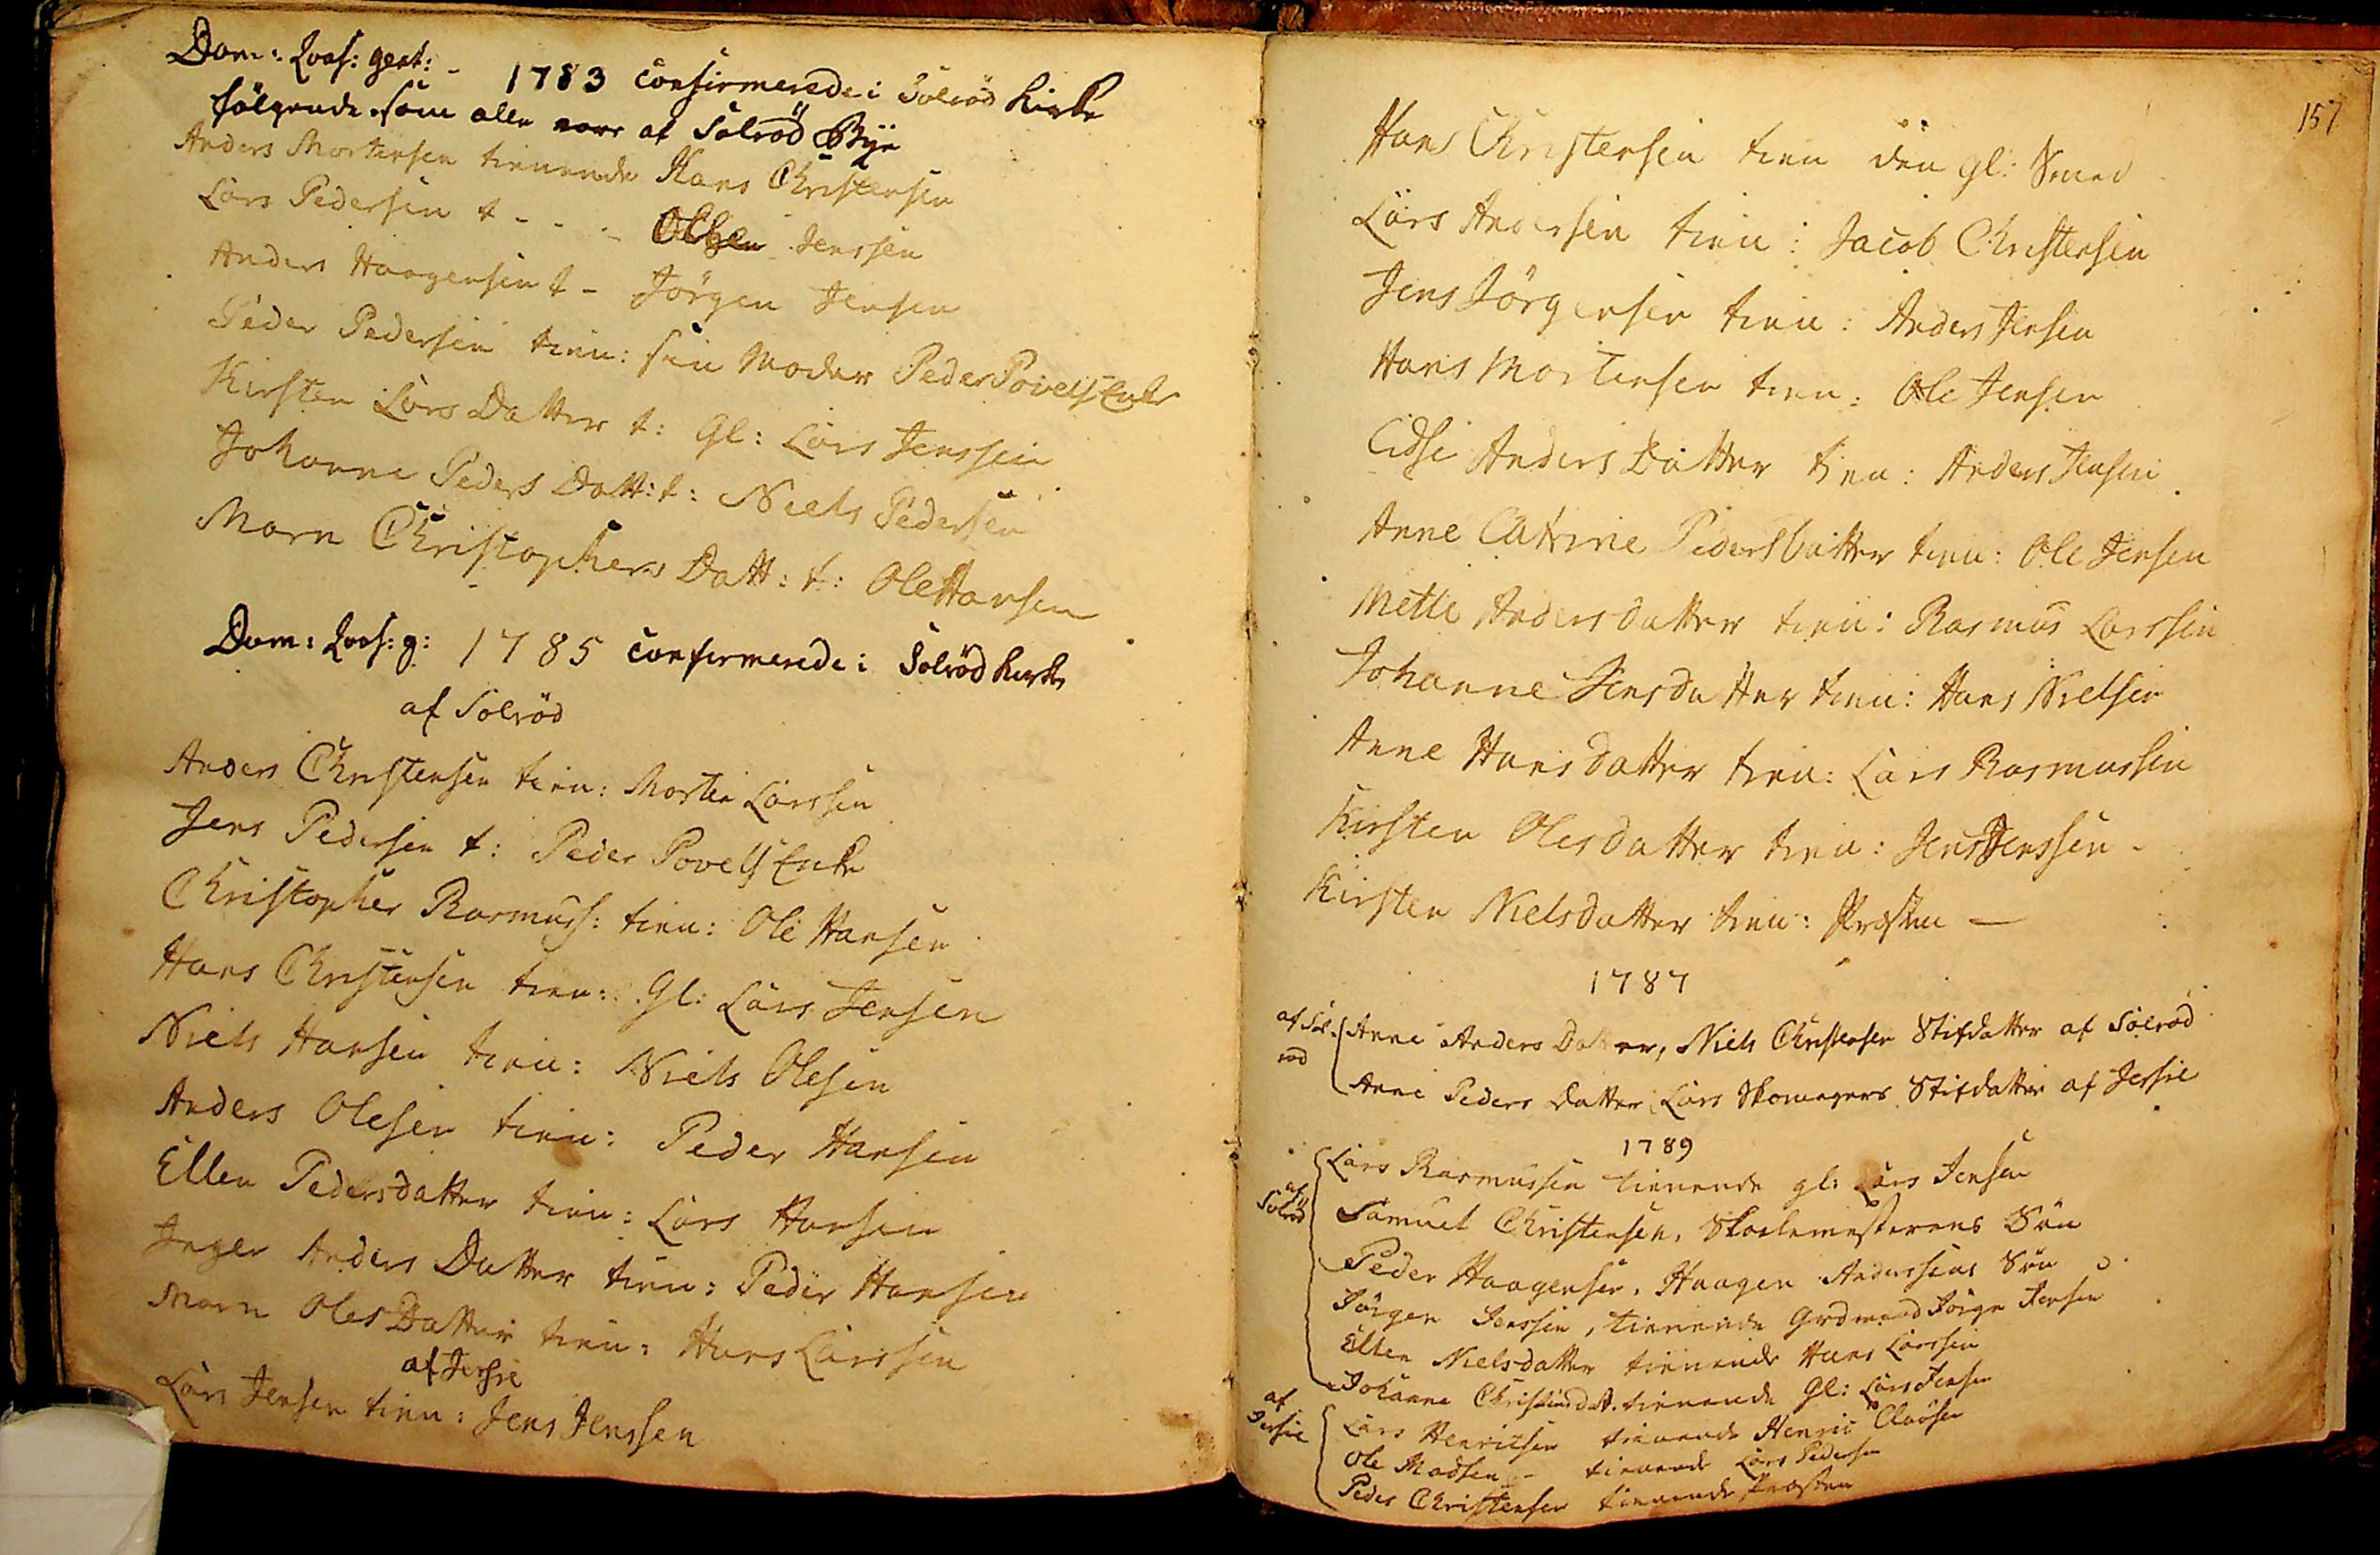Dom: Qvas: gent: - 1783 Confirmerede i Solrød Kirke
følgende som alle vare af Solrød Bye
Anders Mortensen tienende Hans Christensen
Lars Pedersen t - - - Ol## Jenssen
Anders Haagensen t - Jørgen Jensen
Peder Pedersen tien: sin Moder Peder Povels Enke
Kirsten Lars Datter t: Gl: Lars Jenssen
Johanne Peders Datt: t: Niels Pedersen
Marn Christophers Datt: t: Ole Hansen
Dom: qvas:g: 1785 Confirmerede i Solrød Kirke
af Solrød
Anders Christensen tien: Morten Larssen
Jens Pedersen t: Peder Povels Enke
Christopher Rasmuss: tien: Ole Hansen
Hans Christensen tien: Gl: Lars Jensen
Niels Hansen tien: Niels Olesen
Anders Olesen tien: Peder Hansen
Ellen Pedersdatter tien: Lars Hansen
Inger Anders Datter tien: Peder Hansen
Marn Oles Datter tien: Hans Larssen
af Jersie
Lars Jensen tien: Jens Jenssen
157
Hans Christensen tien: den gl: Smed
Lars Andersen tien: Jacob Christensen
Jens Jørgensen tien: Anders Jensen
Hans Mortensen tien: Ole Jensen
Cidse Anders Datter tien: Anders Jensen
Anne Catrine Pedersdatter tien: Ole Jensen
Mette Andersdatter tien: Rasmus Larssen
Johanne Jensdatter tien: Hans Nielsen
Anne Hansdatter tien: Lars Rasmussen
Kirsten Olesdatter tien: Jens Jenssen
Kirsten Nielsdatter tien: Præsten
1787
af Sol¬
rød
Anne Anders Datter, Niels Christensen Stifdatter af Solrød
Anne Peders Datter, Lars Skomagers Stifdatter af Jersie
1789
af
Solrød
Lars Rasmussen tienende gl: Lars Jensen
Samuel Christensen, Skolemesterens Søn
Peder Haagensen, Haagen Andersens Søn
Jørgen Jenssen, tienende Grdmand Jørgn Jensen
Ellen Nielsdatter tienende Hans Larssen
Johanne Christensdatt. tienende gl: Lars Jensen
af
Solrød
Lars Henricsen tienende Henric Clausen
Ole Madsen - tienende Lars Pedersen
Peder Christensen tienende Præsten 
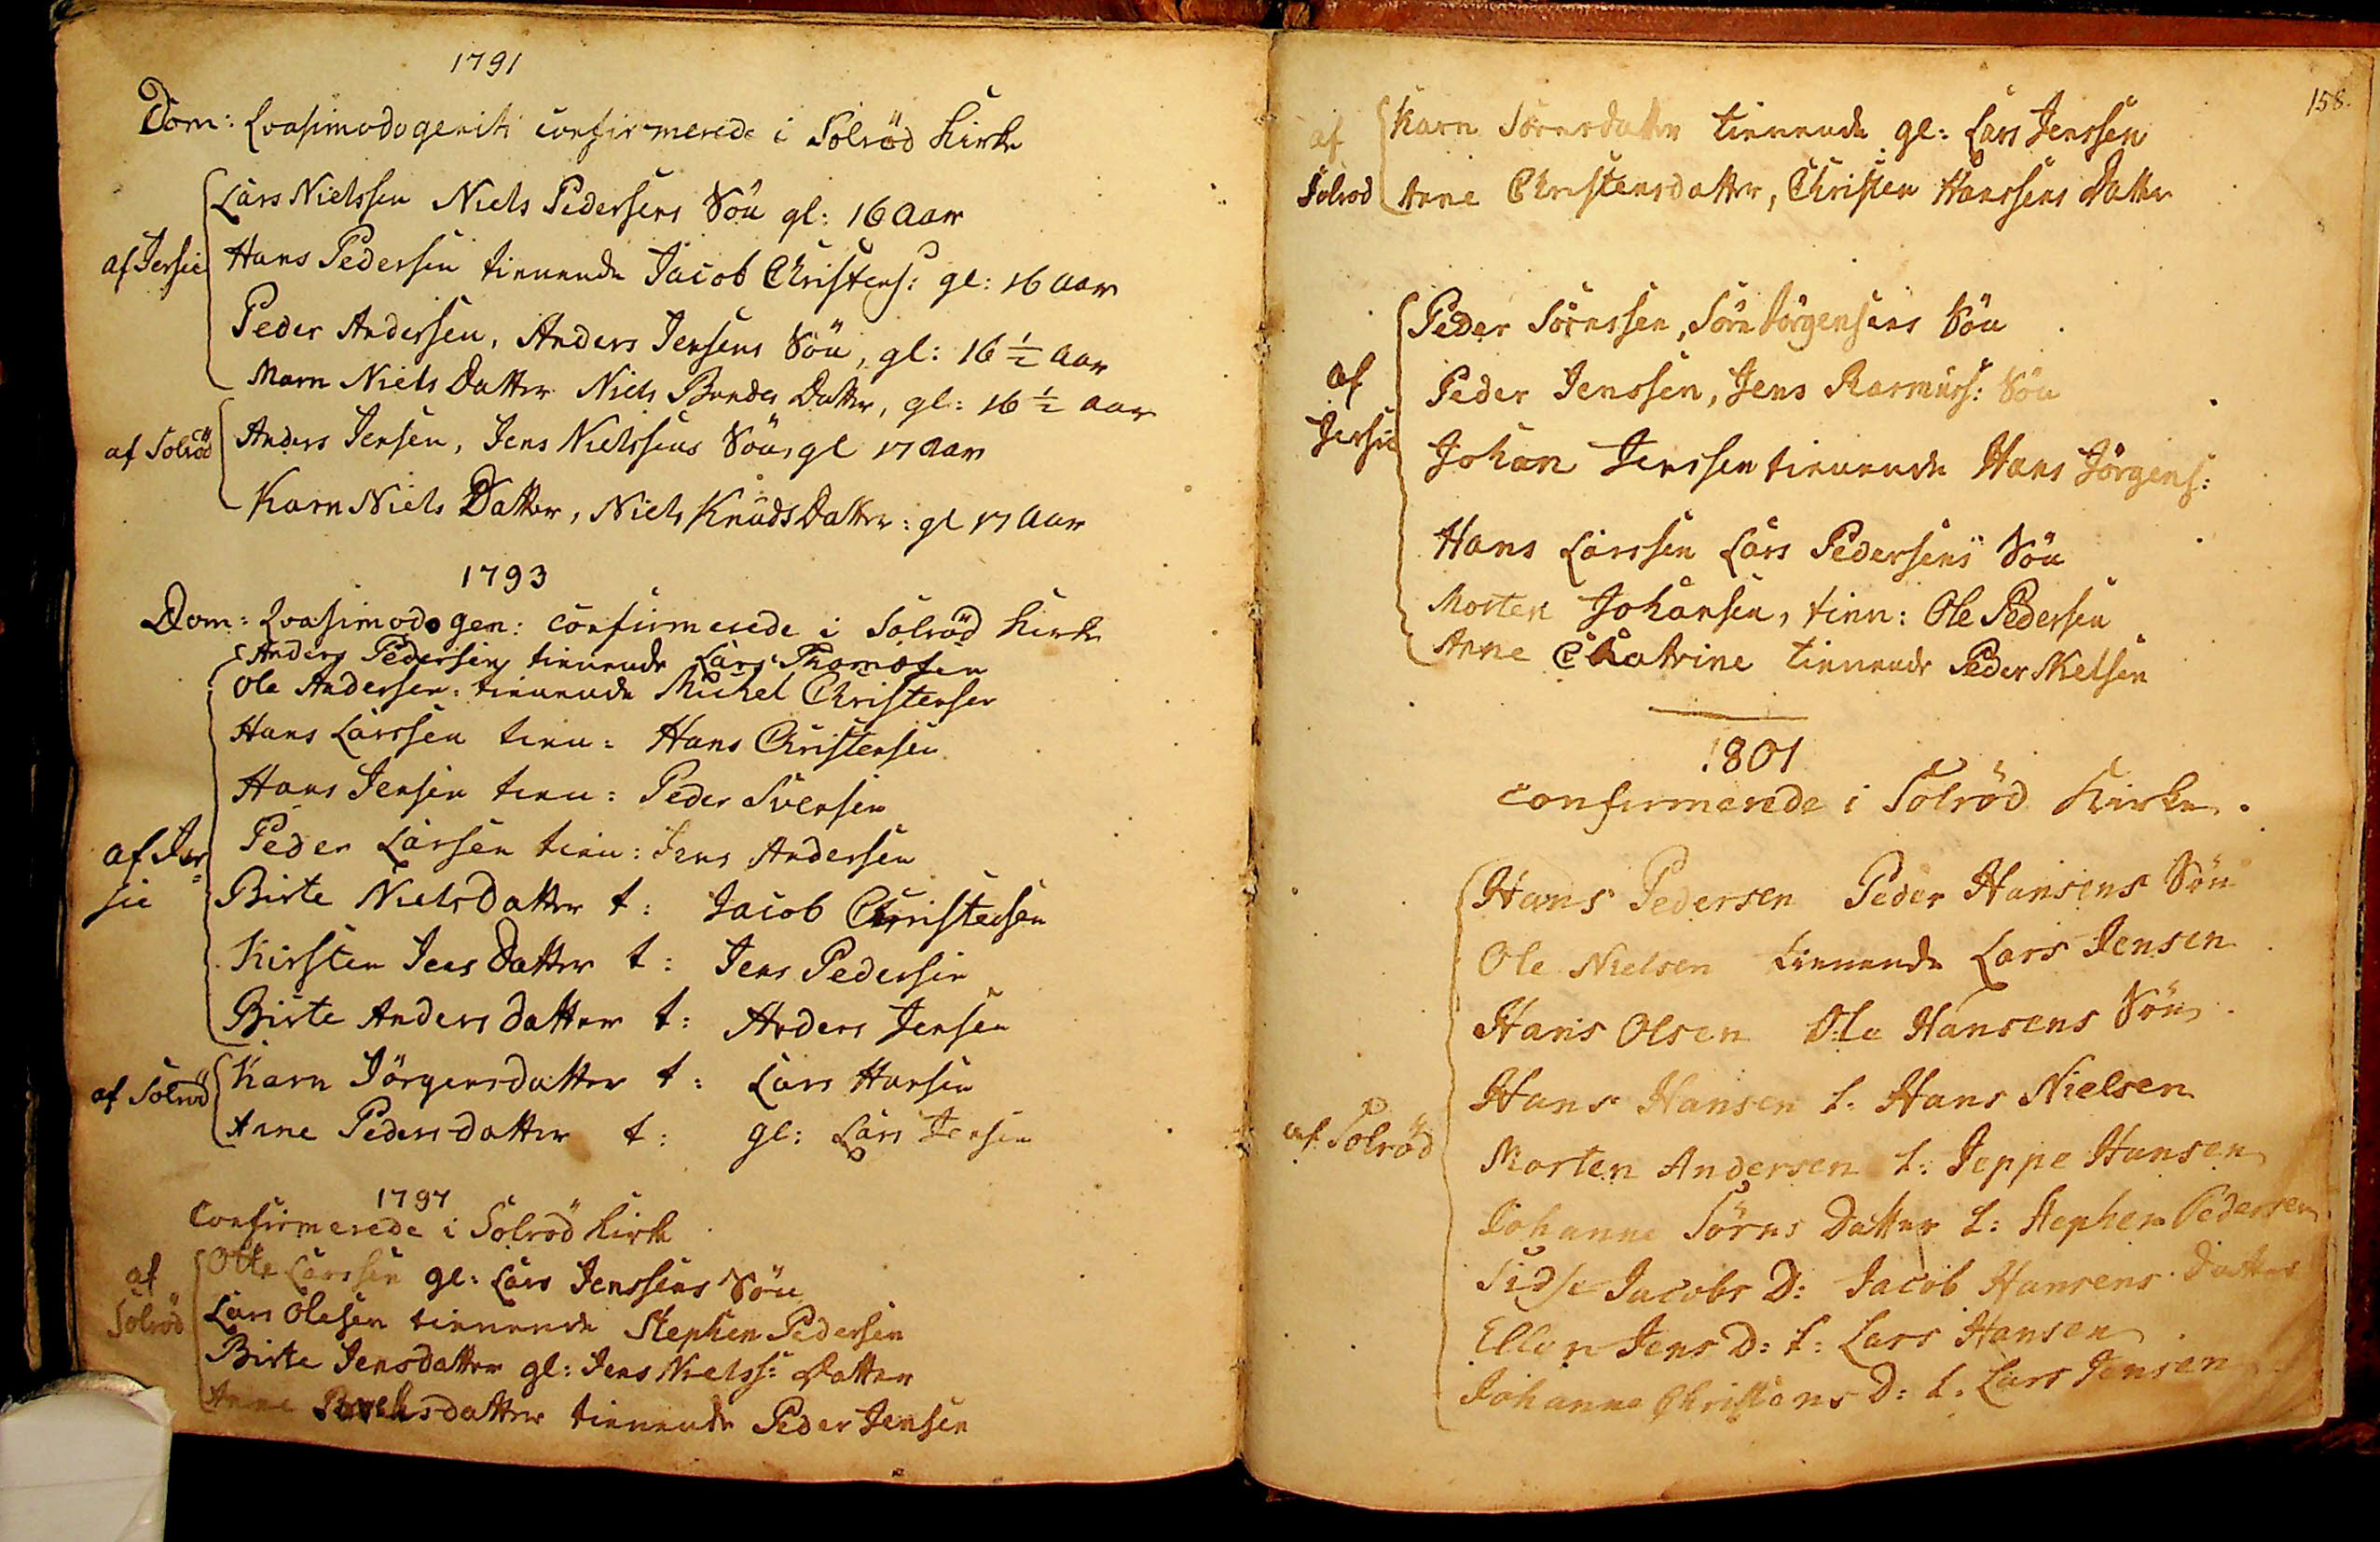1791
Dom: Qvasimodo geniti Confirmerede i Solrød Kirke
Lars Nielssen Niels Pedersens Søn gl: 16 Aar
af Jersie Hans Pedersen tienende Jacob Christens: gl: 16 Aar
Peder Andersen, Anders Jensens Søn, gl: 16½ Aar
Marn Niels Datter Niels Bondes Datter, gl: 16½ Aar
af Solrød Anders Jensen, Jens Nielssens Søn, gl 17 Aar
Karn Niels Datter, Niels Knuds Datter: gl 17 Aar
1793
af Jer¬
sie
Dom: Qvasimodo Gen: Confirmerede i Solrød Kirke
Anders Pedersen, tienende Lars Thomæsen
Ole Andersen, tienende Michel Christensen
Hans Larssen tien: Hans Christensen
Hans Jensen tien: Peder Svensen
Peder Larsen tien: Jens Andersen
Birte Niels Datter t: Jacob Christensen
Kirsten Jens Datter t: Jens Pedersen
Birte Anders Datter t: Anders Jensen
af Solrød Karn Jørgensdatter t: Lars Hansen
Anne Pedersdatter t: gl: Lars Jensen
1797
af
Solrød
Confirmerede i Solrød Kirke
Otte Larssen gl: Lars Jenssens Søn
Lars Olesen tienende Stephen Pedersen
Birte Jensdatter gl: Jens Nielss: Datter
Anne Povelsdatter## tienende Peder Jensen
158.
af
Solrød
Karn Sørnsdatter tienende gl: Lars Jenssen
Anne Christensdatter, Christen Hanssens Datter
af
Jersie
Peder Sørnssen, Sørn Jørensens Søn
Peder Jenssen, Jens Rasmuss: Søn
Johan Jenssen tienende Hans Jørgens:
Hans Larssen Lars Pedersens Søn
Morten Johansen, Tien: Ole Pedersen
Anne Chatrine tienende Peder Nielsen
1801
Confirmerede i Solrød Kirke.
Hans Pedersen Peder Hansens Søn
Ole Nielsen tienende Lars Jensen
Hans Olsen Ole Hansens Søn
Hans Hansen t: Hans Nielsen
af Solrød Morten Andersen t: Jeppe Hansen
Johanne Sørns Datter t: Stephen Pedersen
Sidse Jacobs D: Jacob Hansens Datter
Ellen Jens D: t: Lars Hansen
Johanne Christens D: t: Lars Jensen.